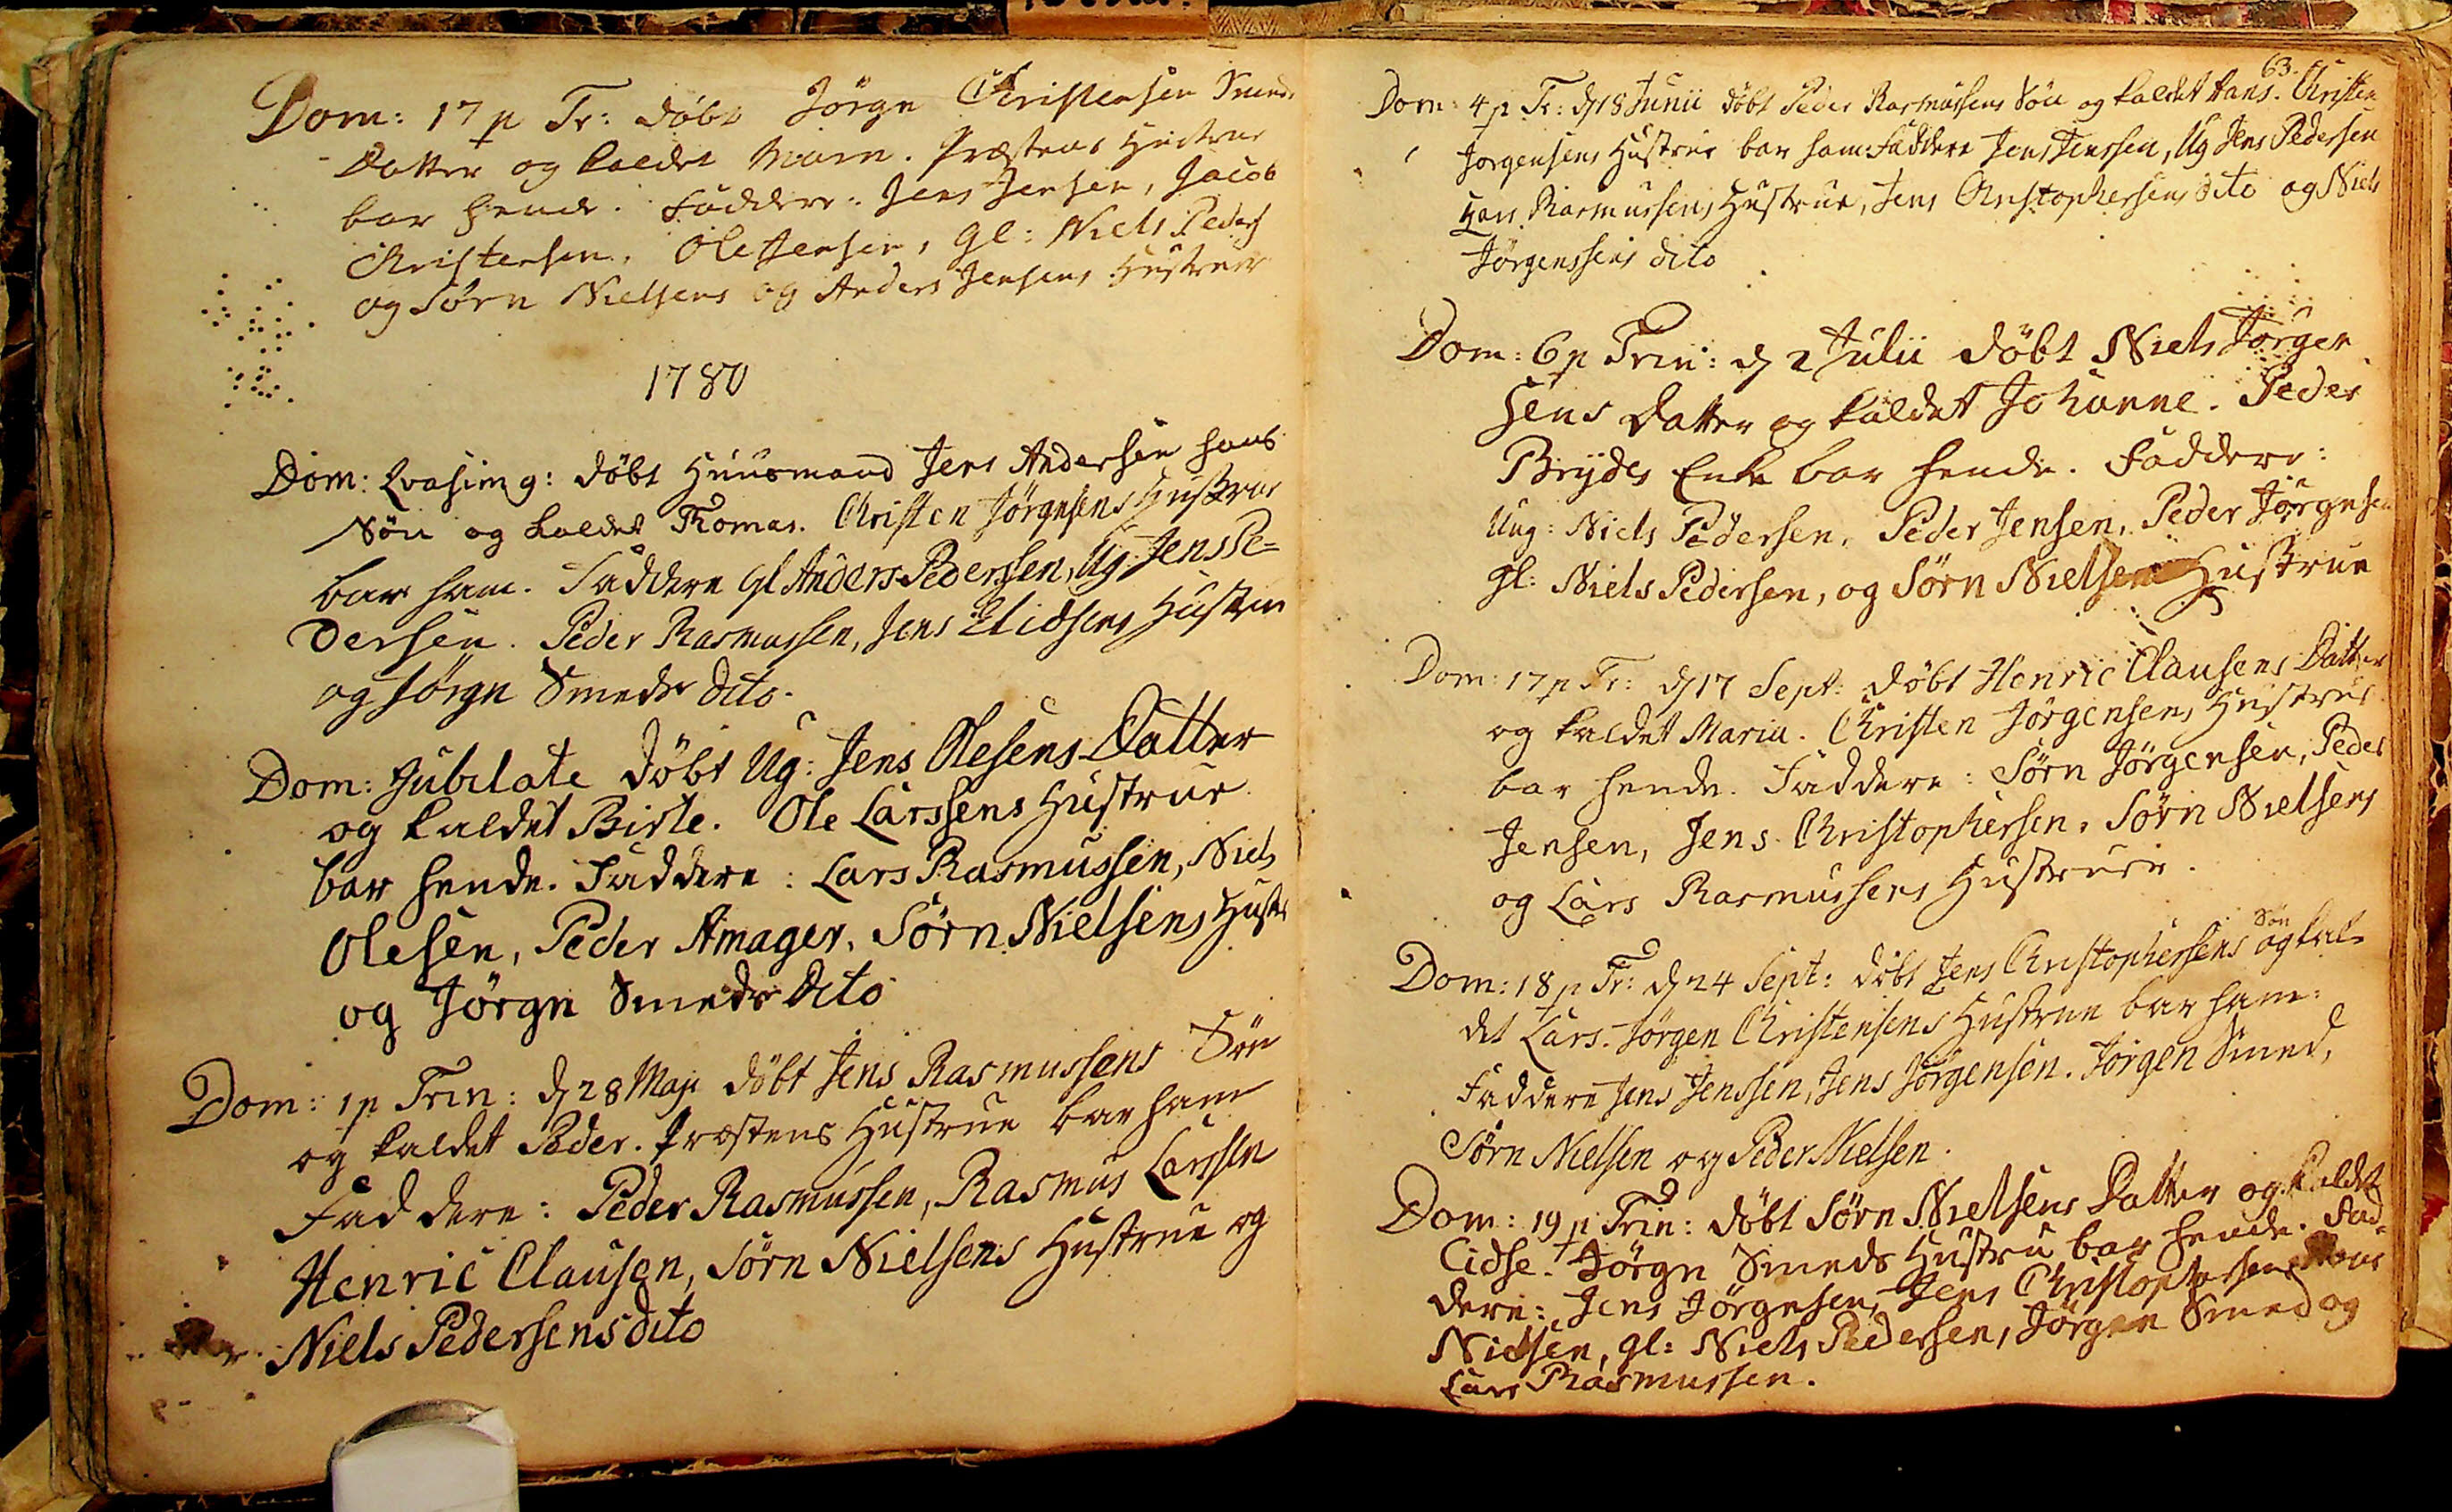Dom: 17 p Tr: døbt Jørgn Christensen Smeds
Datter og kaldet Marn. Præstens Hustrue
bar hende. Faddere: Jens Jensen, Jacob
Christensen, Ole Jensen, gl: Niels Peders
og Sørn Nielsens og Anders Jensens Hustruer
1780
Dom: Qvasimg: døbt Huusmand Jens Andersen hans
Søn og kaldet Thomas. Christen Jørgnsens Hustrue
bar ham. Faddere gl Anders Pederssen, Ug: Jens Pe¬
dersen. Peder Rasmussen, Jens Eliæsens Hustrue
og Jørgn Smeds dito   
Dom: Jubilate døbt Ug: Jens Olesens Datter
og kaldet Birte. Ole Larssens Hustrue
bar hende. Faddere: Lars Rasmussen, Niels
Olesen, Peder Amager, Sørn Nielsens Hustr
og Jørgn Smeds dito
Dom: 1 p Trin: d 28 Maji døbt Jens Rasmussens Søn
og kaldet Peder. Præstens Hustrue bar ham
Faddere: Peder Rasmussen, Rasmus Larssen
Henric Clausen; Sørn Nielsens Hustrue og 
Niels Pedersens dito
63.
Dom: 4 p Tr: d 18 Junii døbt Peder Rasmussens Søn og kaldet Hans. Christen
Jørgensens Hustrue bar ham. Faddere Jens Jenssen, Ug Jens Pedersen
Lars Rasmussens Hustrue, Jens Christophersens dito og Niels
Jørgensens dito.
Dom: 6 p Trin: d 2 Julii døbt Niels Jørgen
sens Datter og kaldet Johanne. Peder
Brydes Enke bar hende. Faddere:
Ung: Niels Pedersen, Peder Jensen, Peder Jørgnsen
gl: Niels Pedersen, og Sørn Nielsens Hustrue
Dom: 17 p Tr: d 17 Sept: døbt Henric Clausens Datter
og kaldet Maria. Christen Jørgensens Hustrue
bar hende. Faddere: Sørn Jørgensen, Peder
Jensen, Jens Christophersen, Sørn Nielsens
og Lars Rasmussens Hustruer.
Søn
Dom: 18 p Tr: d 24 Sept: døbt Jens Christophersens og kal
det Lars. Jørgen Christensens Hustrue bar ham:
Faddere Jens Jenssen, Jens Jørgensen. Jørgen Smed,
Sørn Nielsen og Peder Nielsen.
Dom: 19 p Trin: døbt Sørn Nielsens Datter og kaldet
Cidse. Jørgn Smeds Hustru bar hende. Fad¬
dere: Jens Jørgnsen, Jens Christophersen, Hans
Nielsen, gl: Niels Pedersen, Jørgen Smed og
Lars Rasmussen.
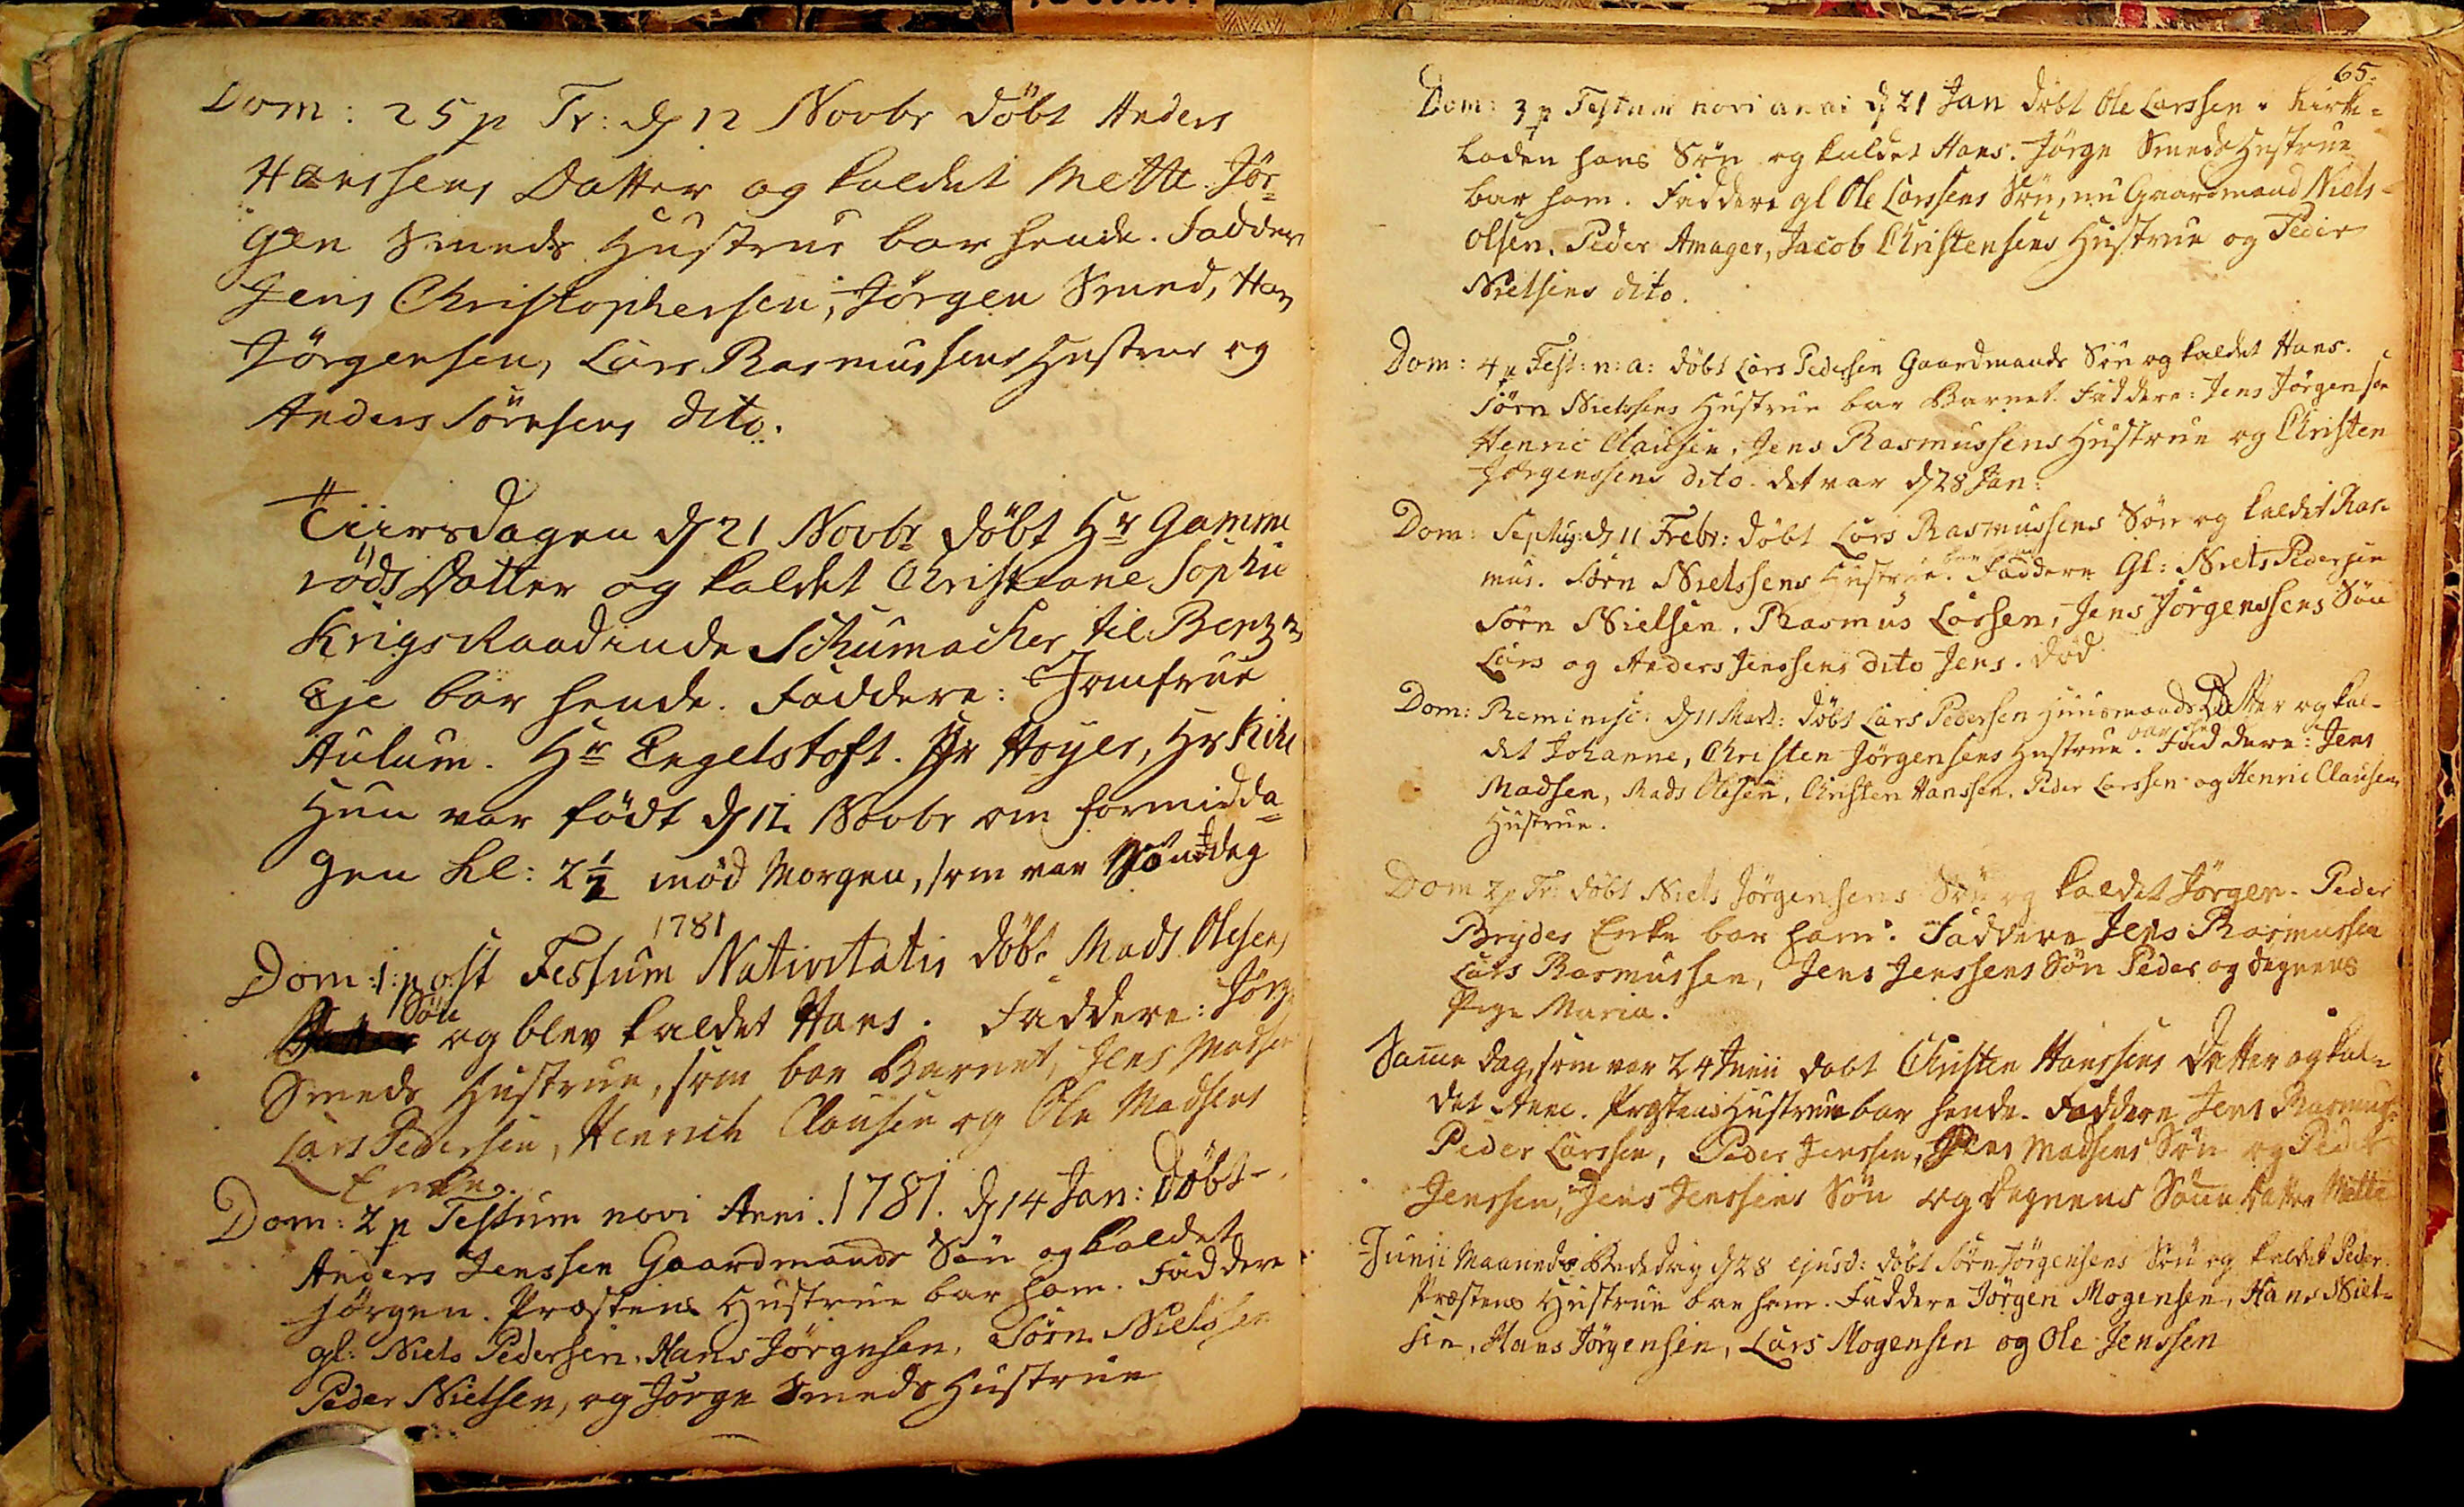Dom: 25 p Tr: d 12 Novbr døbt Anders
Hanssens Datter og kaldet Mette. Jør¬
gen Smeds Hustrue bar hende. Faddere
Jens Christophersen, Jørgen Smed, Hans
Jørgensen, Lars Rasmussens Hustrue og 
Anders Sørnsens dito.
Tiirsdagen d 21 Novbr døbt Hr Gamme
røds Datter og kaldet Christiane Sophie
KrigsRaadinde Schumacher til Benzon
Eje bar hende. Faddere: Jomfrue
Aulum. Hr Engelstoft. Hr Høyer, Hr Kihl
Hun var født d 12 Novbr om formidda¬
gen kl: 2½ mod Morgen, som var Sønddag
1781
Dom: 1: post Festum Nativitatis døbt Mads Olesens
Søn
og blev kaldet Hans. Faddere: Jørgn
Smeds Hustrue, som bar Barnet, Jens Madsen
Lars Pedersen, Henrick Clausen og Ole Madsens
Enke.
Dom: 2 p Festum novi Anni. 1781. d 14 Jan: døbt
Anders Jenssen Gaardmands Søn og kaldet
Jørgen. Præstens Hustrue bar ham. Faddere
gl: Niels Pedersen, Hans Jørgnsen, Sørn Nielssen
Peder Nielsen, og Jørgn Smeds Hustrue
65.
Dom: 3 p Festum novi anni d 21 Jan døbt Ole Larssen i Kirke¬
Laden hans Søn og kaldet Hans. Jørgn Smeds Hustrue
bar ham. Faddere gl Ole Larssens Søn, nu Gaardmand Niels
Olsen, Peder Amager, Jacob Christensens Hustrue og Peder
Nielsens dito.
Dom: 4 p Fest: n: a: døbt Lars Pedersen Gaardmands Søn og kaldet Hans.
Sørn Nielssens Hustrue bar Barnet. Faddere: Jens Jørgensen
Henric Clausen, Jens Rasmussens Hustrue og Christen
Jørgensens dito. det var d 28 Jan:
Dom: Septug: d 11 Febr: døbt Lars Rasmussens Søn og kaldet Ras¬
bar ham
mus. Sørn Nielssens Hustrue. Faddere Gl: Niels Pedersen
Sørn Nielsen. Rasmus Larsen, Jens Jørgenssens Søn
Lars og Anders Jenssens dito Jens. død
Dom: Reminisc: d 11 Mart: døbt Lars Pedersen Huusmands Datter og kal¬
bar hende
det Johanne, Christen Jørgensens Hustrue. Faddere: Jens
Madsen, Mads Olesen, Christen Hanssen, Peder Larssen og Henric Clausens
Hustrue.
Dom: 2p Tr: døbt Niels Jørgensens Søn og kaldet Jørgen. Peder
Brydes Enke bar ham. Faddere Jens Rasmussen
Lars Rasmussen, Jens Jenssens Søn Peder og Degnens
Pige Maria.
Same Dag, som var 24 Junii døbt Christen Hanssens Datter og kal¬
det Anne. Præstens Hustrue bar hende. Faddere Jens Rasmus:
Peder Larssen, Peder Jenssen, Jens Madsens Søn og Peder
Jenssen, Jens Jenssens Søn og Degnens Sønne Datter Mette
Junii Maaneds Bededag d 28 ejusd: døbt Sørn Jørgensens Søn og kaldet Peder
Præstens Hustrue bar ham. Faddere Jørgen Mogensen. Hans Niel¬
sen, Hans Jørgensen, Lars Mogensen og Ole Jenssen